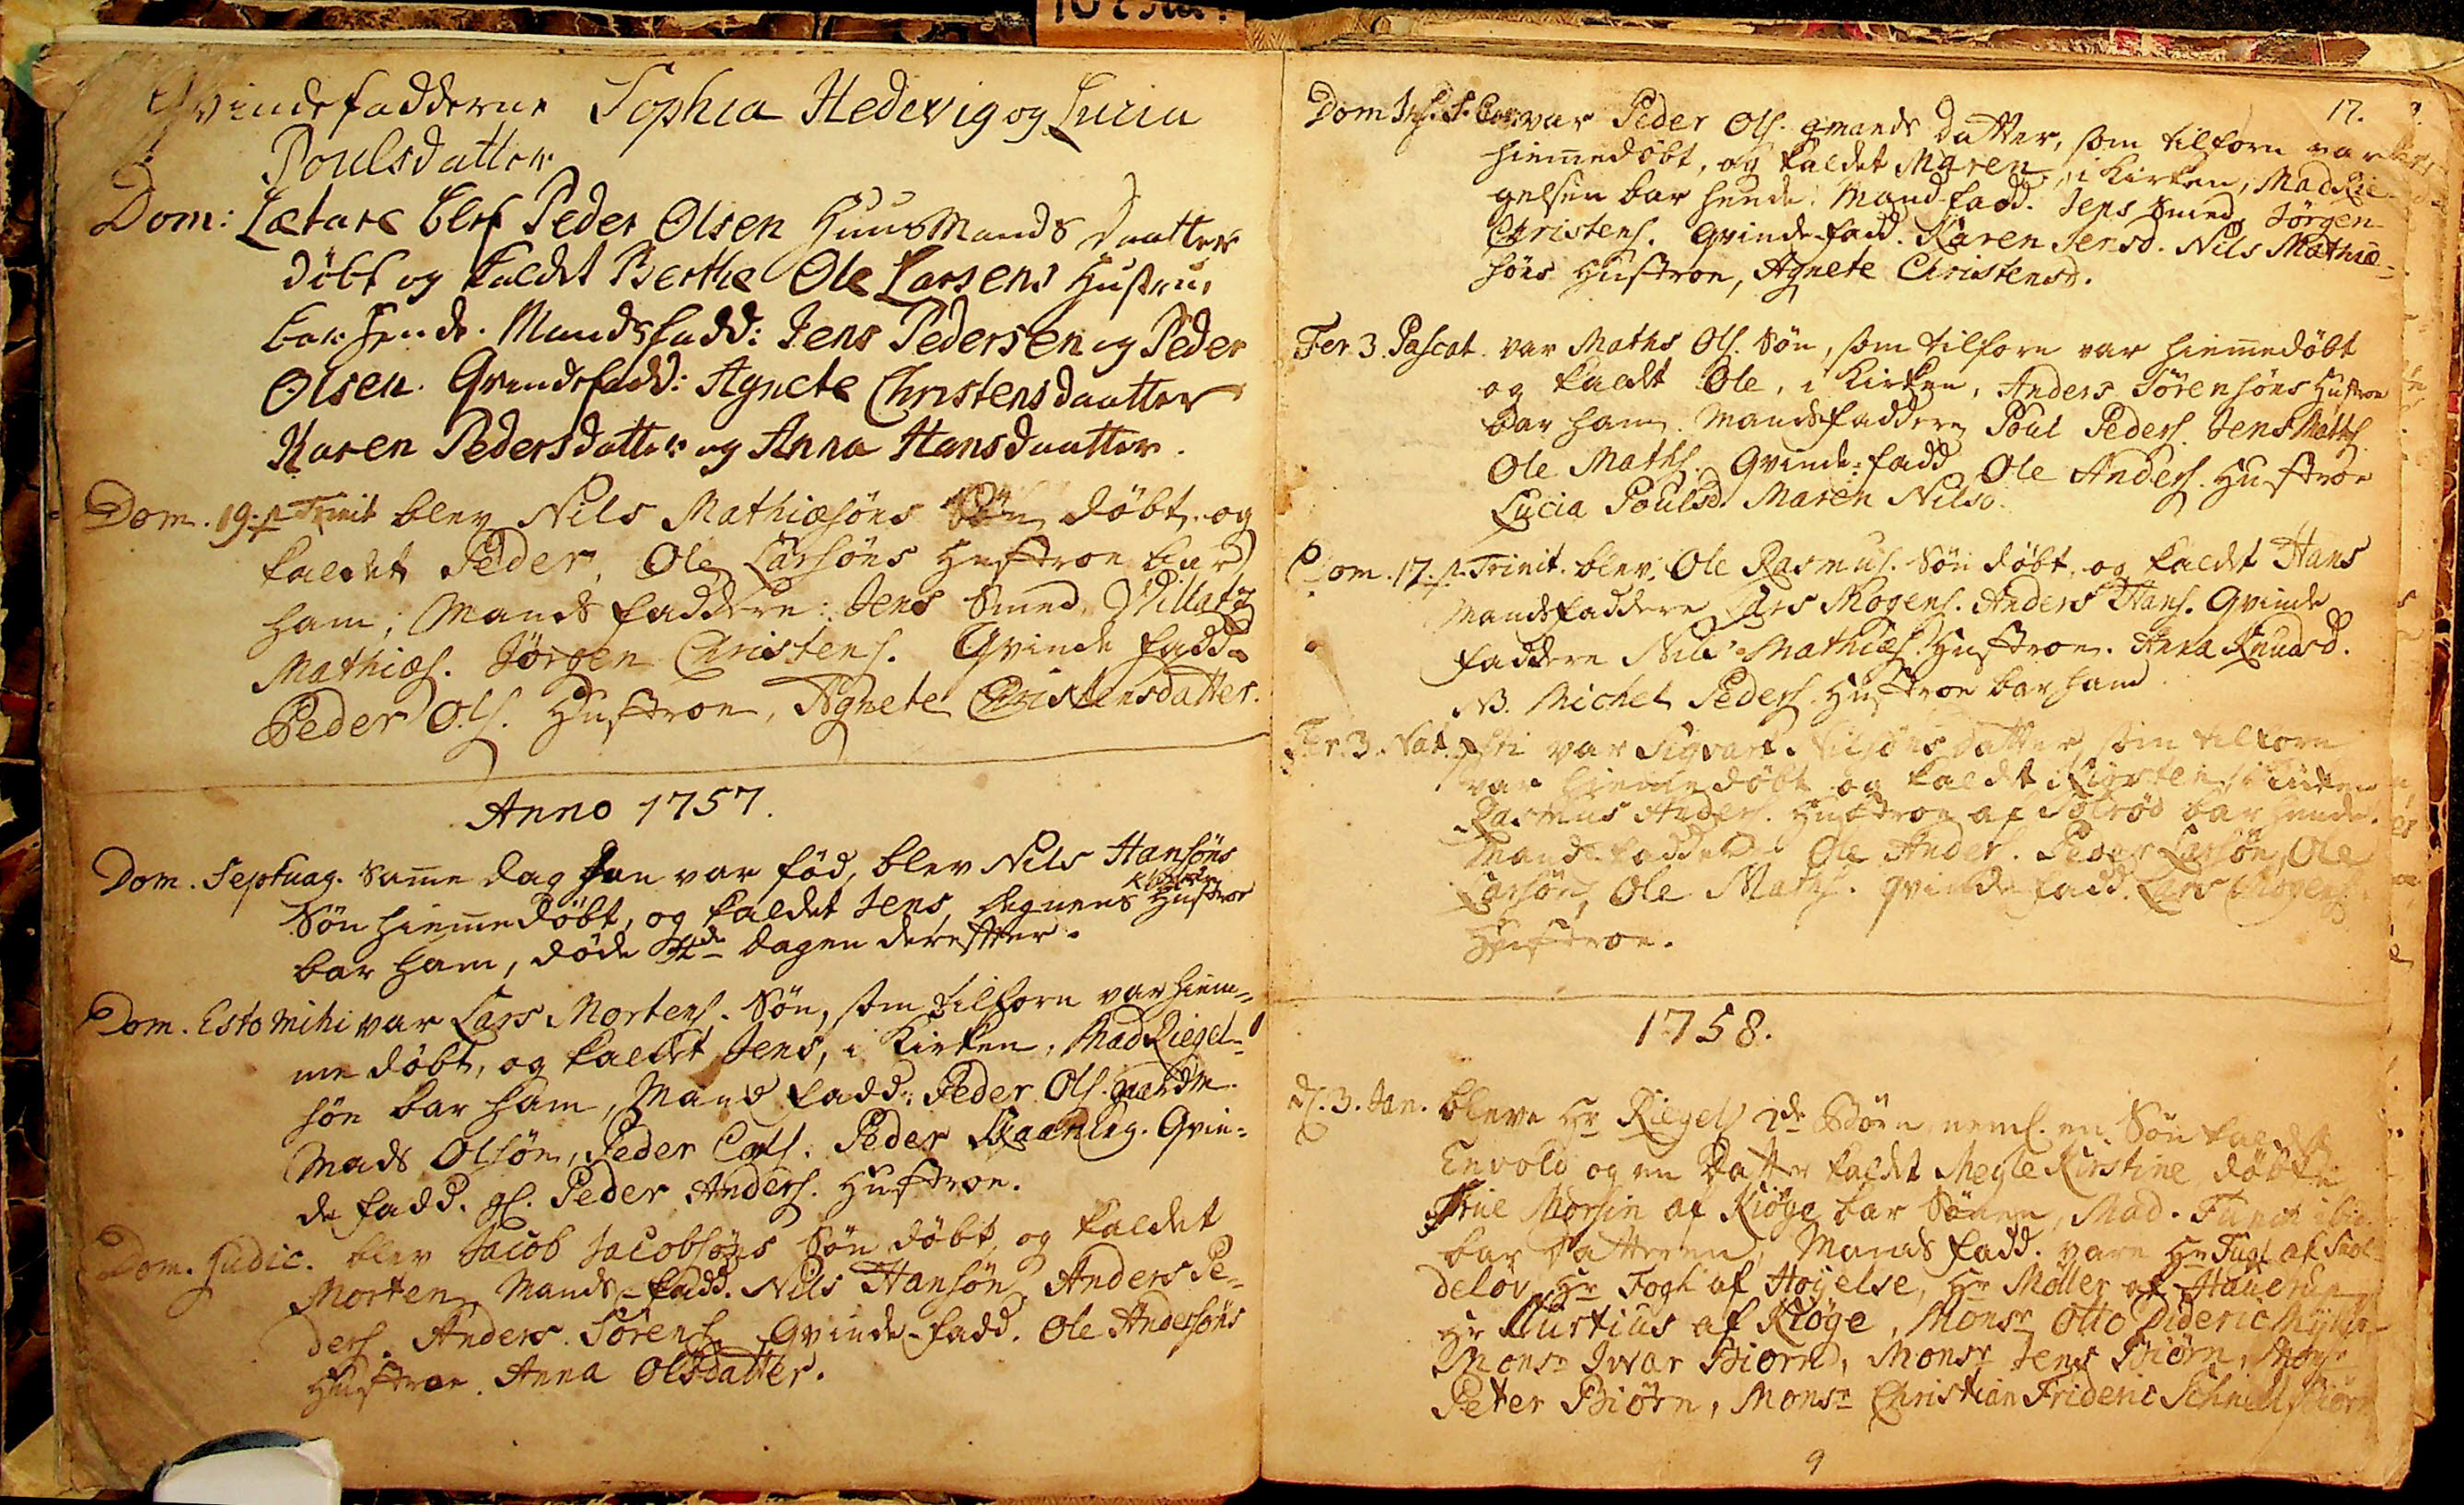Qvindefadderne Sophia Hedevig og Lucia
Poulsdatter
Dom: Lætare blef Peder Olsen Huusmands daatter
døbt og kaldet Berthe Ole Larsens Hustrue
bar hende. Mandsfadd: Jens Pedersen og Peder
Olsen. Qvindefadd: Agnete Christensdaatter
Karen Pedersdatter og Anna Hansdaatter.
Dom.19. p Trinit blev Nils Mathiæsøns Søn døbt og
kaldet Peder, Ole Larsøns Hustroe bar
ham, Mands faddere: Jens Smed, Willatz
Mathiæs. Jørgen Christens. Qvinde fadd.
Peder Ols. Hustroe, Agnete Christensdatter
Anno 1757.
Dom. Septuag. Same dag han var fød, blev Nils Hansøns
Klixbøls
Søn Hiemmedøbt, og kaldet Jens, Degnens Hustroe
bar ham, døde 4de Dagen derefter.
Dom. Esto Mihi var Lars Mortens. Søn, som tilforn var hiem¬
medøbt, og kaldet Jens, i Kirken. Mad Riegel¬
søn bar ham, Mand fadd: Peder Ols. gaardm.
Mads Olsøn, Peder Carls. Peder Skaaning. Qvin¬
de fadd. gl. Peder Anders. Hustroe. 
Dom. Judic. blev Jacob Jacobsøns Søn døbt, og kaldet
Morten, Mand-fadd. Niels Hansøn, Anders Pe¬
ders. Anders Sørens. Qvinde-fadd. Ole Andersøns
Hustroe. Anna Olsdatter.
17.
Dom Inh. S. Pas##: var Peder Ols. gmands Datter, som tilforn var
hiemmedøbt, og kaldet Maren, i Kirken, Mad Rie¬
gelsen bar hende. Mand-fadd. Jens Smed, Jørgen
Christens. Qvind-fadd. Karen Jensd. Nils Mathiæ¬
søns Hustroe, Agnete Christensd.
Fer. 3. Pascat. var Maths Ols. Søn, som tilforn var hiemmedøbt
og kaldt Ole, i Kirken, Anders Sørensøns Hustroe
bar ham. Mandsfaddere Poul Peders. Jens Maths.
Ole Maths. Qvinde-fadd Ole Anders. Hustroe
Lucia Poulsd. Maren Nilsd.
Dom. 17. p. Trinit. blev Ole Rasmus. Søn døbt og kaldet Hans
Mandsfaddere Lars Mogens. Anders Hans. Qvinde
faddere Nils Mathiæs. Hustroe. Anna Knudsd.
NB. Michel Peders. Hustroe bar ham.
Fer. 3. Nat. Xsti var Sigvart Nilsøns Datter, som tilforn
var hiemmedøbt og kaldt Kirsten, i Kirken
Rasmus Anders. Hustroe af Solrød bar hende.
Mands-faddere Ole Anders. Peder Larsøn, Ole
Larsøn, Ole Maths. qvinde-fadd. Lars Mogens.
Hustroe
1758.
d. 3. Jan. bleve Hr. Riegels 2de Børn, neml. en Søn kaldet
Envold og en Datter kaldt Megle Kirstine, døbte,
Frue Morsin af Kiøge bar Sønnen, Mad. Funch ibid.
bar Datteren, Mands fadd. vare Hr Fugl af Snol¬
deløv, Hr Fogh af Høyelse, Hr Møller af Haudrup,
Hr Curtius af Kiøge, Monsr Otto Dideric Myller,
Monsr Iwar Biørn, Monsr Jens Biørn, Monsr
Peter Biørn, Monsr Christian Frideric Schnell Biørn
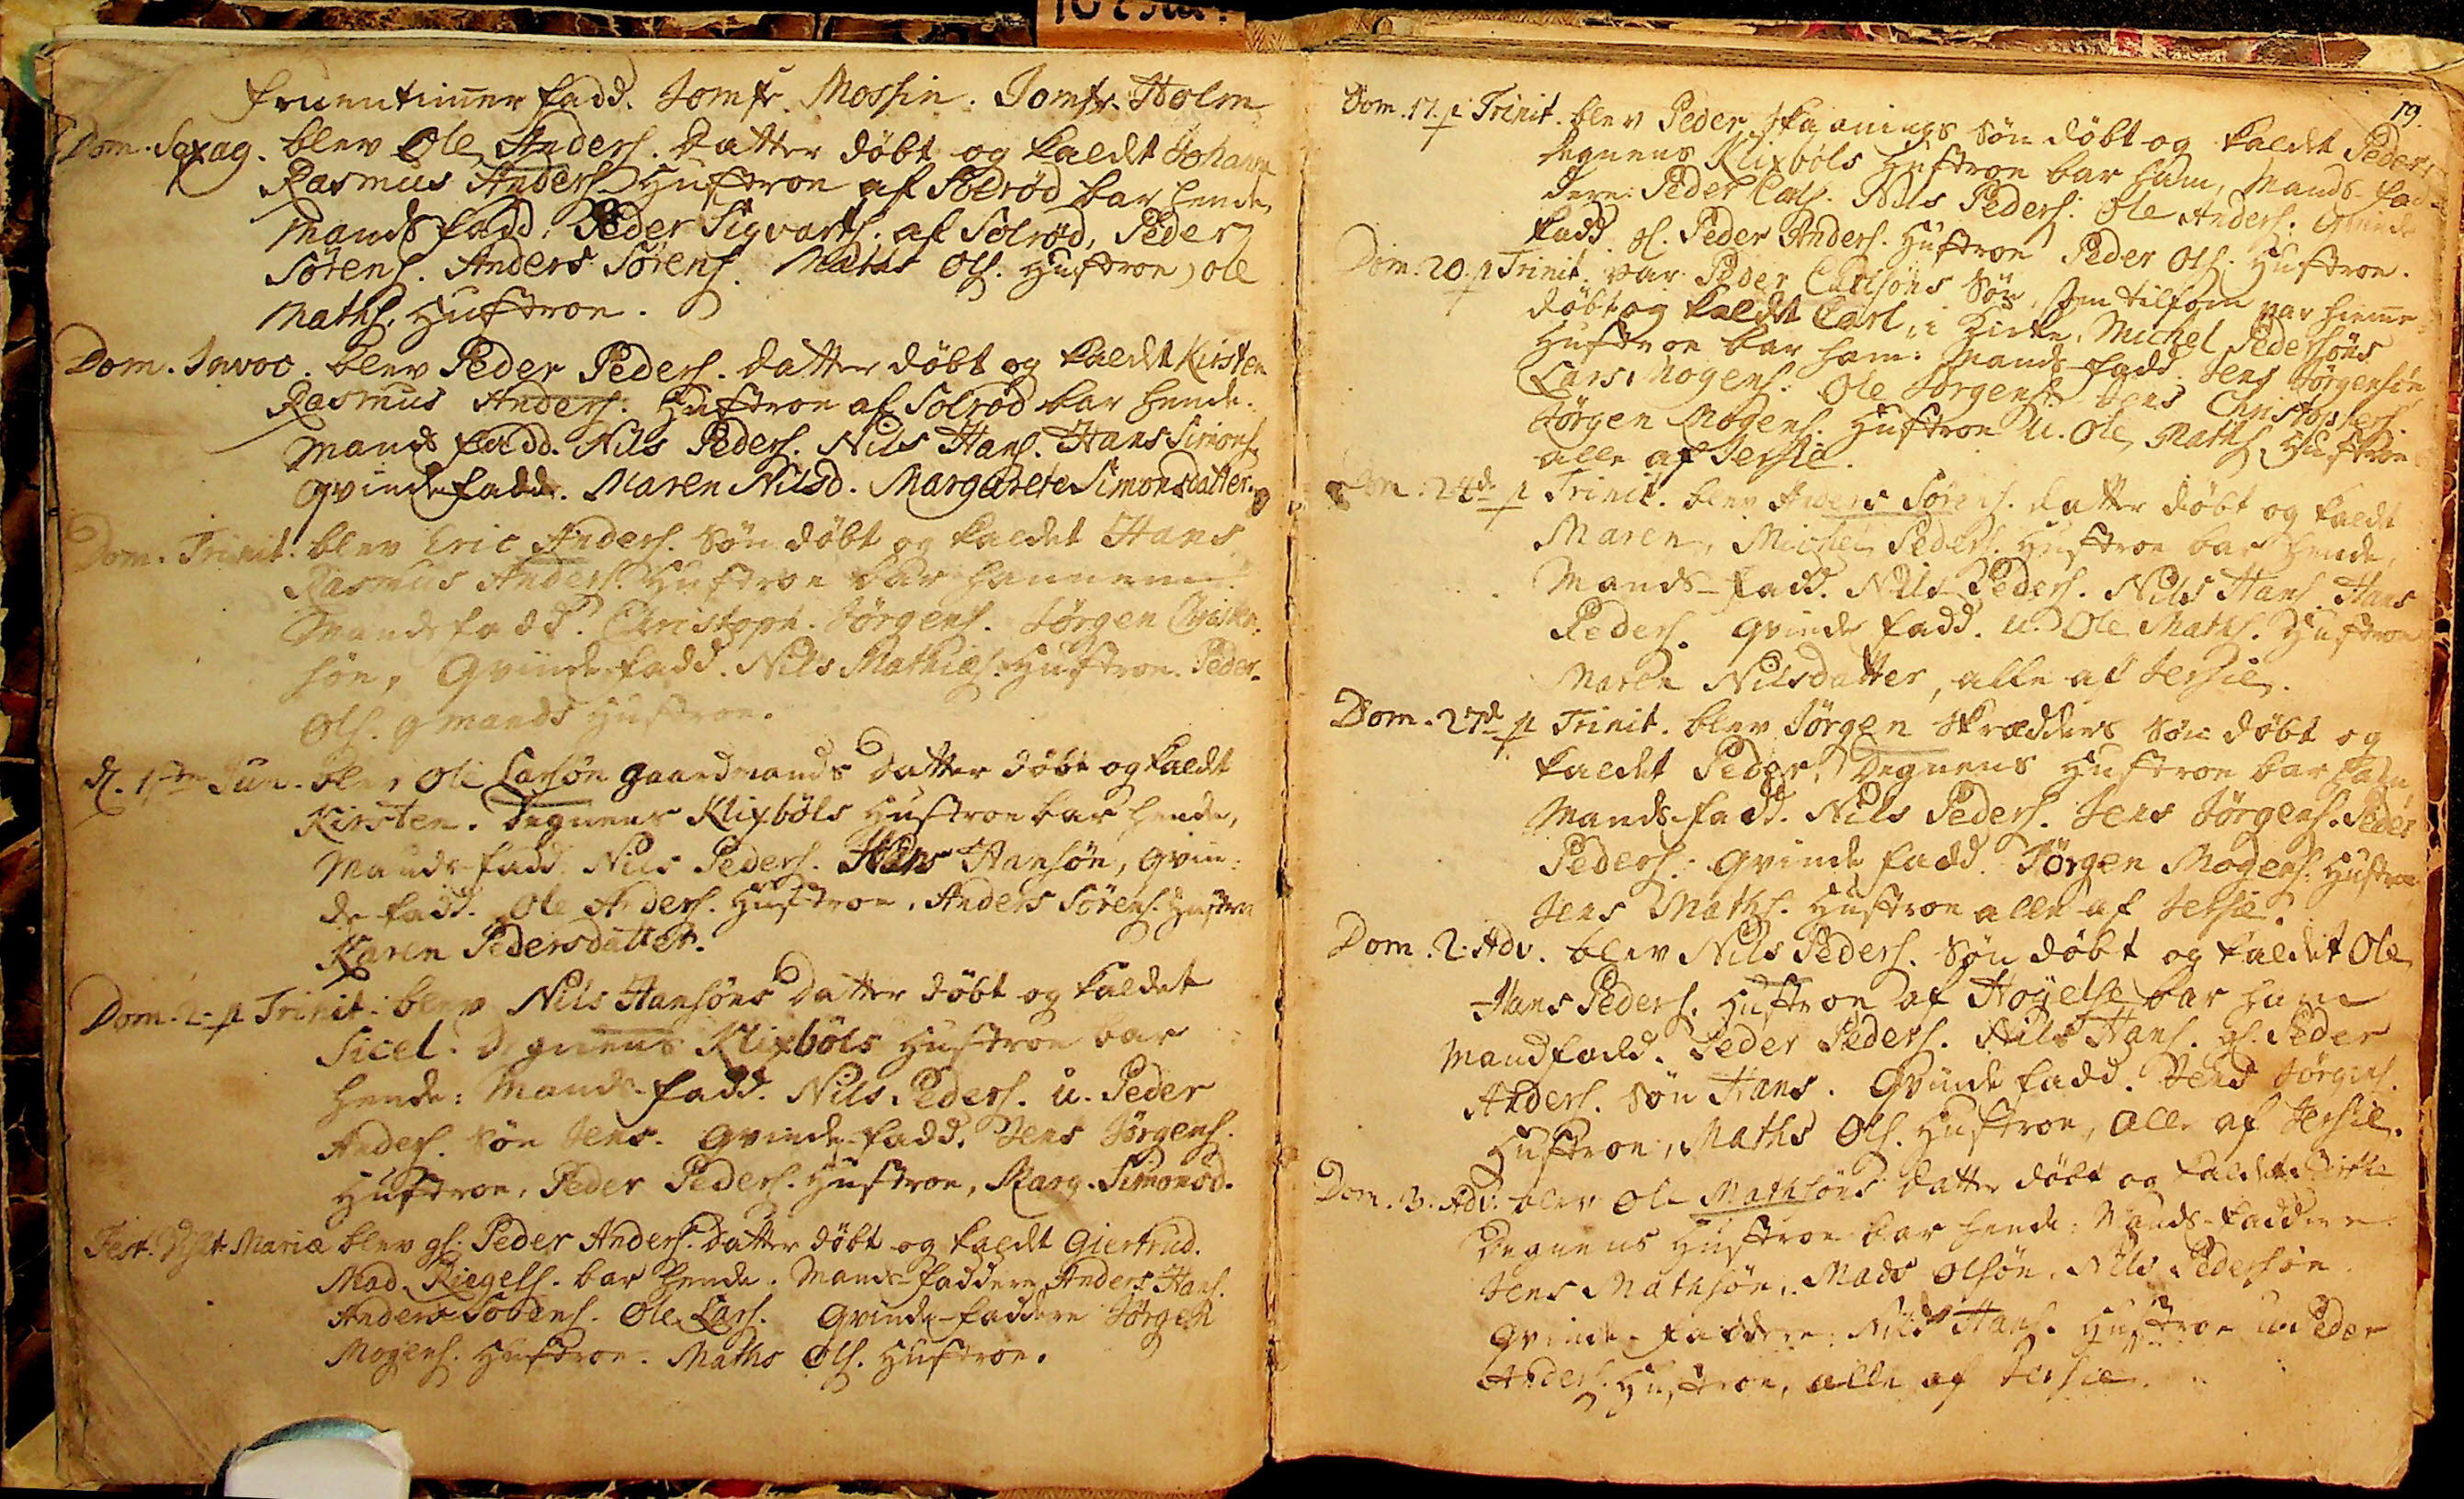fruentimer fadd. Jomfr. Mossin. Jomfr. Holm
Dom. Sexag. blev Ole Anders. Datter døbt og kaldt Johanne
Rasmus Anders. Hustroe af Solrød bar hende,
Mands fadd, Peder Sigvarts. af Solrød, Peder
Sørens. Anders Sørens. Maths Ols. Hustroe, Ole
Maths. Hustroe.
Dom Invoc. blev Peder Peders. Datter døbt og kaldt Kirsten
Rasmus Anders. Hustroe af Solrød bar hende.
Mands fadd Nils Peders. Nils Hans. Hans Simons.
Qvindefadd. Maren Nilsd. Margarete Simonsdatter.
Dom. Trinit blev Eric Anders. Søn døbt og kaldet Hans
Rasmus Anders. Hustroe bar hannem.
Mandsfadd. Christoph. Jørgens. Jørgen Christen¬
søn, Qvinde-fadd. Nils Mathiæs. Hustroe. Peder
Ols. gmands Hustroe.
d. 1ste Jun. blev Ole Larsøn Gaardmands Datter døbt og kaldt
Kirsten. Degnens Klixbøls Hustroe bar hende,
Mands-fadd: Nils Peders. Hans Hansøn, Qvin¬
defadd. Ole Anders. Hustroe. Anders Sørens. Hustroe
Karen Pedersdatter.
Dom. 2. p Trinit. blev Nils Hansøns Datter døbt og kaldet
Sicel. Degnens Klixbøls Hustroe bar
hende: Mands-fadd. Nils Peders. u. Peder
Anders. Søn Jens. Qvinde-fadd. Jens Jørgens.
Hustroe, Peder Peders. Hustroe, Marg. Simonsd.
Fest. Visit Mariæ blev gl. Peder Anders. Datter døbt og kaldt Giertrud.
Mad Riegels. bar hende. Mands-Faddere Anders Hans.
Anders Sørens. Ole Lars. Qvinde-faddere Jørgen
Mogens. Hustroe. Maths Ols. Hustroe.
19.
Dom. 17. p Trinit. blev Peder Skaanings Søn døbt og kaldt Peder,
Degnens Klixbøls Hustroe bar ham, Mands-fad¬
dere: Peder Carls. Nils Peders: Ole Anders. Qvinde
fadd. gl. Peder Anders. Hustroe Peder Ols. Hustroe.
Dom. 20. p Trinit. var Peder Carlsøns Søn, som tilforn var hiemme¬
døbt og kaldt Carl, i Kirke. Michel Pedersøns
Hustroe bar ham. Mands-fadd. Jens Jørgensøn,
Lars Mogens: Ole Jørgens. Jens Christophers
Jørgen Mogens. Hustroe U. Ole Maths. Hustroe
alle af Jersie.
Dom: 24de p Trinit. blev Anders Sørens. Datter døbt og kaldt
Maren, Michel Peders. Hustroe bar hende,
Mands-fadd. Nils Peders. Nils Hans. Hans
Peders. Qvinde fadd. u. Ole Maths. Hustroe
Maren Nilsdatter, alle af Jersie.
Dom. 27de p Trinit. blev Jørgen Skrædders Søn døbt og
kaldet Peder. Degnens Hustroe bar ham,
Mands-fadd. Nils Peders. Jens Jørgens. Peder
Peders: Qvinde fadd. Jørgen Mogens: Hustroe
Jens Maths. Hustroe alle af Jersie.
Dom. 2. Adv. blev Nils Peders. Søn døbt og kaldet Ole
Hans Peders. Hustroe af Høyelse bar ham
Mandfadd. Peder Peders. Nils Hans. gl. Peder
Anders. Søn Hans. Qvinde fadd. Jens Jørgens.
Hustroe, Maths Ols. Hustroe, Alle af Jersie.
Dom. 3. Adv. blev Ole Mathsøns Datter døbt og kaldet Birthe
Degnens Hustroe bar hende: Mands-faddere
Jens Mathsøn, Mads Olsøn, Nils Pedersøn.
Qvinde-faddere: Nils Hans. Hustroe u. Peder
Anders. Hustroe, alle af Jersie.